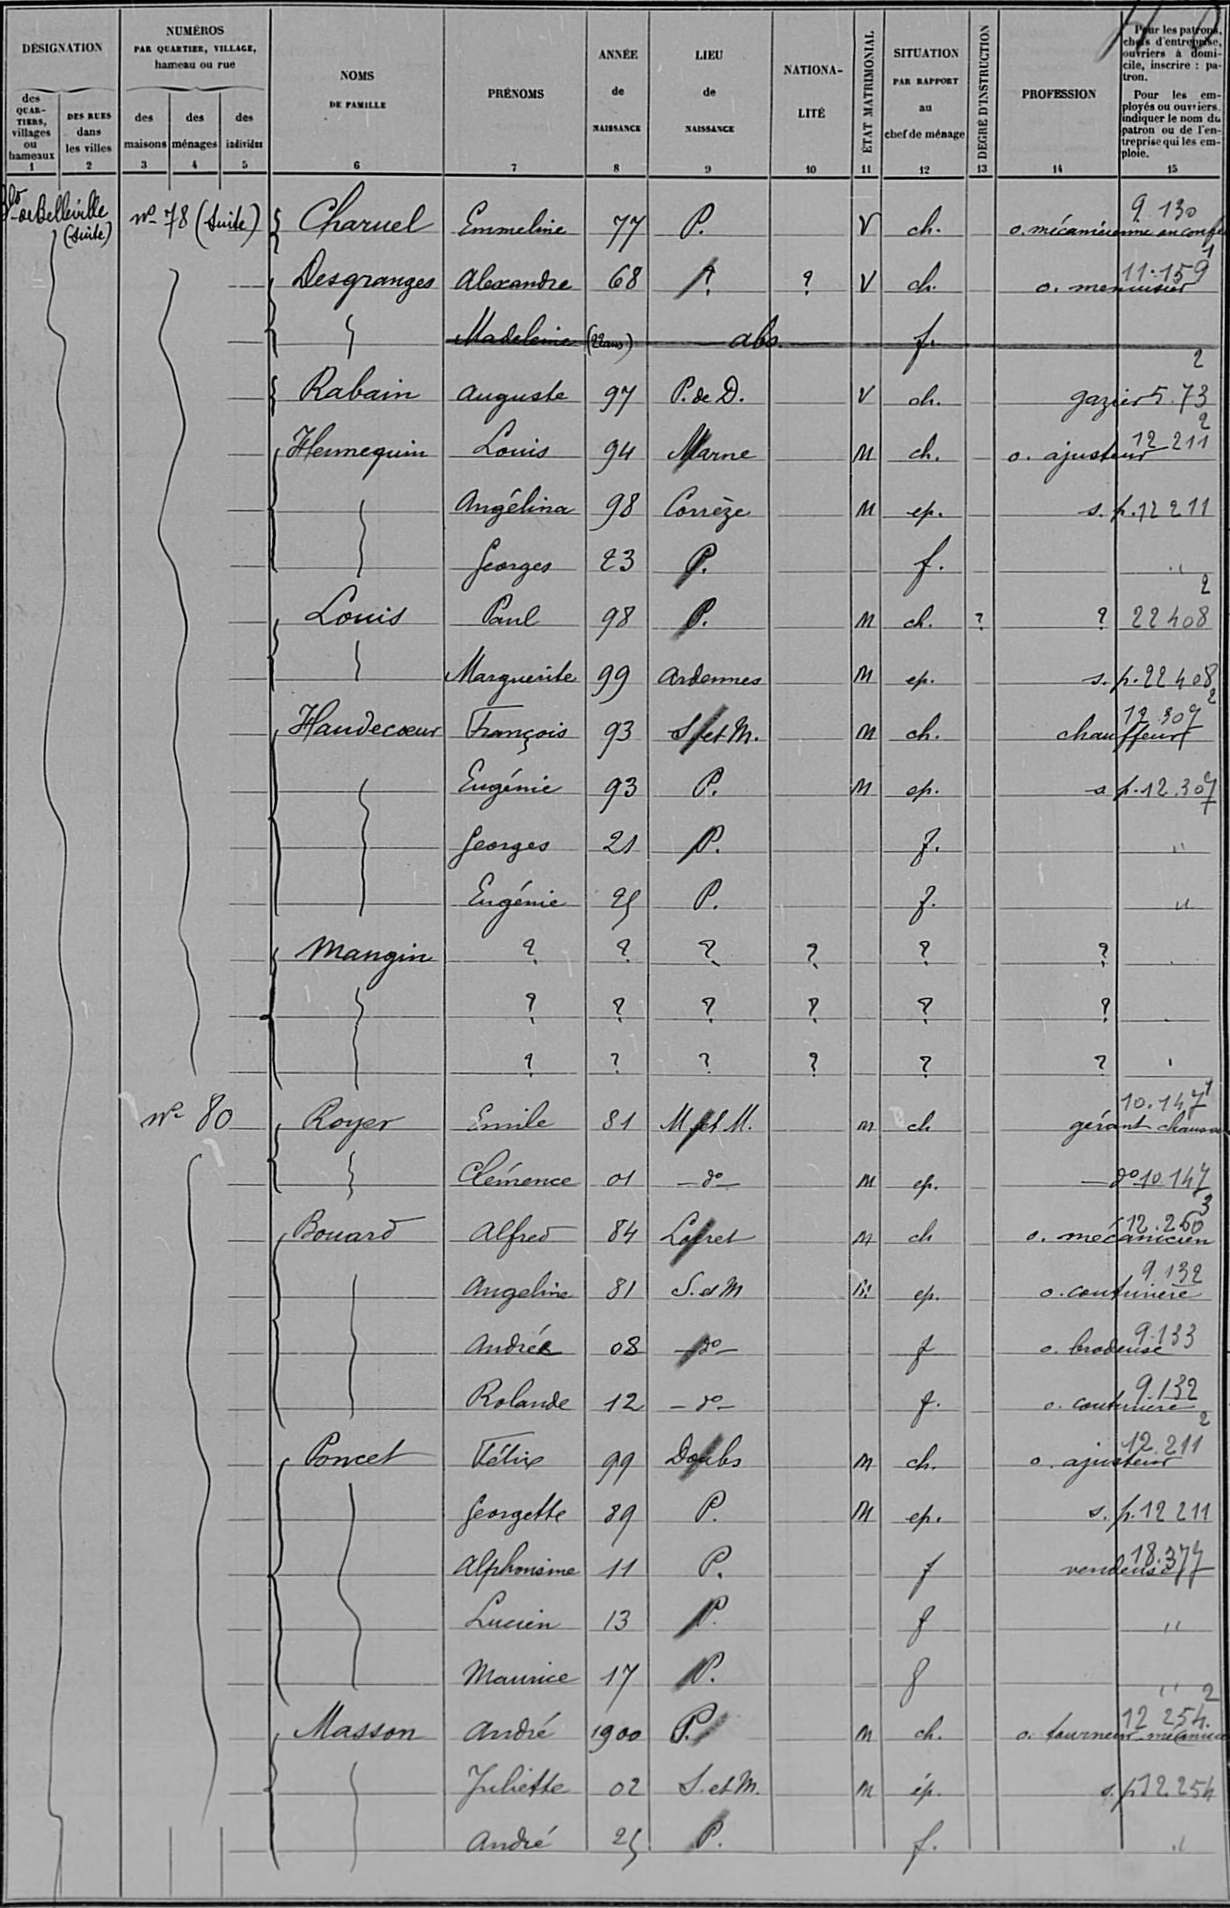Charuel/Emmeline/77/P /¤/V/ch /¤/o mécanicienne au conf/¤
Desgranges/Alexandre/68/¤/¤/V/ch /¤/o menuisier/¤
¤/Madeleine/(22 ans)/-abs-/¤/¤/f /¤/¤/¤
Rabain/Auguste/97/P de D /¤/V/ch /¤/gazier/573
Hennequin/Louis/94/Marne/¤/M/ch /¤/o ajusteur/¤
¤/Angéline/98/Corrèze/¤/M/ep /¤/s p /12211
¤/Georges/23/P /¤/¤/f /¤/¤/¤
Louis/Paul/98/P /¤/M/ch /¤/¤/22408
¤/Marguerite/99/Ardennes/¤/M/ep /¤/s p /22408
Haudecoeur/François/93/S et M /¤/M/ch /¤/chauffeur/¤
¤/Eugénie/93/P /¤/M/ep /¤/s p /12307
¤/Georges/21/P /¤/¤/f /¤/¤/¤
¤/Eugénie/25/P /¤/¤/f /¤/¤/¤
Mangin/¤/¤/¤/¤/¤/¤/¤/¤/¤
¤/¤/¤/¤/¤/¤/¤/¤/¤/¤
¤/¤/¤/¤/¤/¤/¤/¤/¤/¤
Royer/Emile/81/M et M /¤/m/ch/¤/gérant chauss/¤
¤/Clémence/01/d°/¤/M/ep /¤/d°/10147
Bouard/Alfred/84/Loiret/¤/M/ch/¤/o mécanicien/¤
¤/Angeline/81/S et M /¤/M/ep /¤/o couturière/9132
¤/Andrée/08/d°/¤/¤/f/¤/o brodeuse/¤
¤/Rolande/12/d°/¤/¤/f /¤/o couturière/¤
Poncet/Félix/99/Doubs/¤/M/ch /¤/o ajusteur/¤
¤/Georgette/89/P /¤/M/ep /¤/s p /12211
¤/Alphonsine/11/P /¤/¤/f/¤/vendeuse/¤
¤/Lucien/13/P /¤/¤/f/¤/¤/¤
¤/Maurice/17/P /¤/¤/f/¤/¤/¤
Masson/André/1900/P /¤/M/ch /¤/o tourneur mécanicien/¤
¤/Juliette/02/S et M /¤/M/ép /¤/s p /12254
¤/André/25/P /¤/¤/f /¤/¤/¤